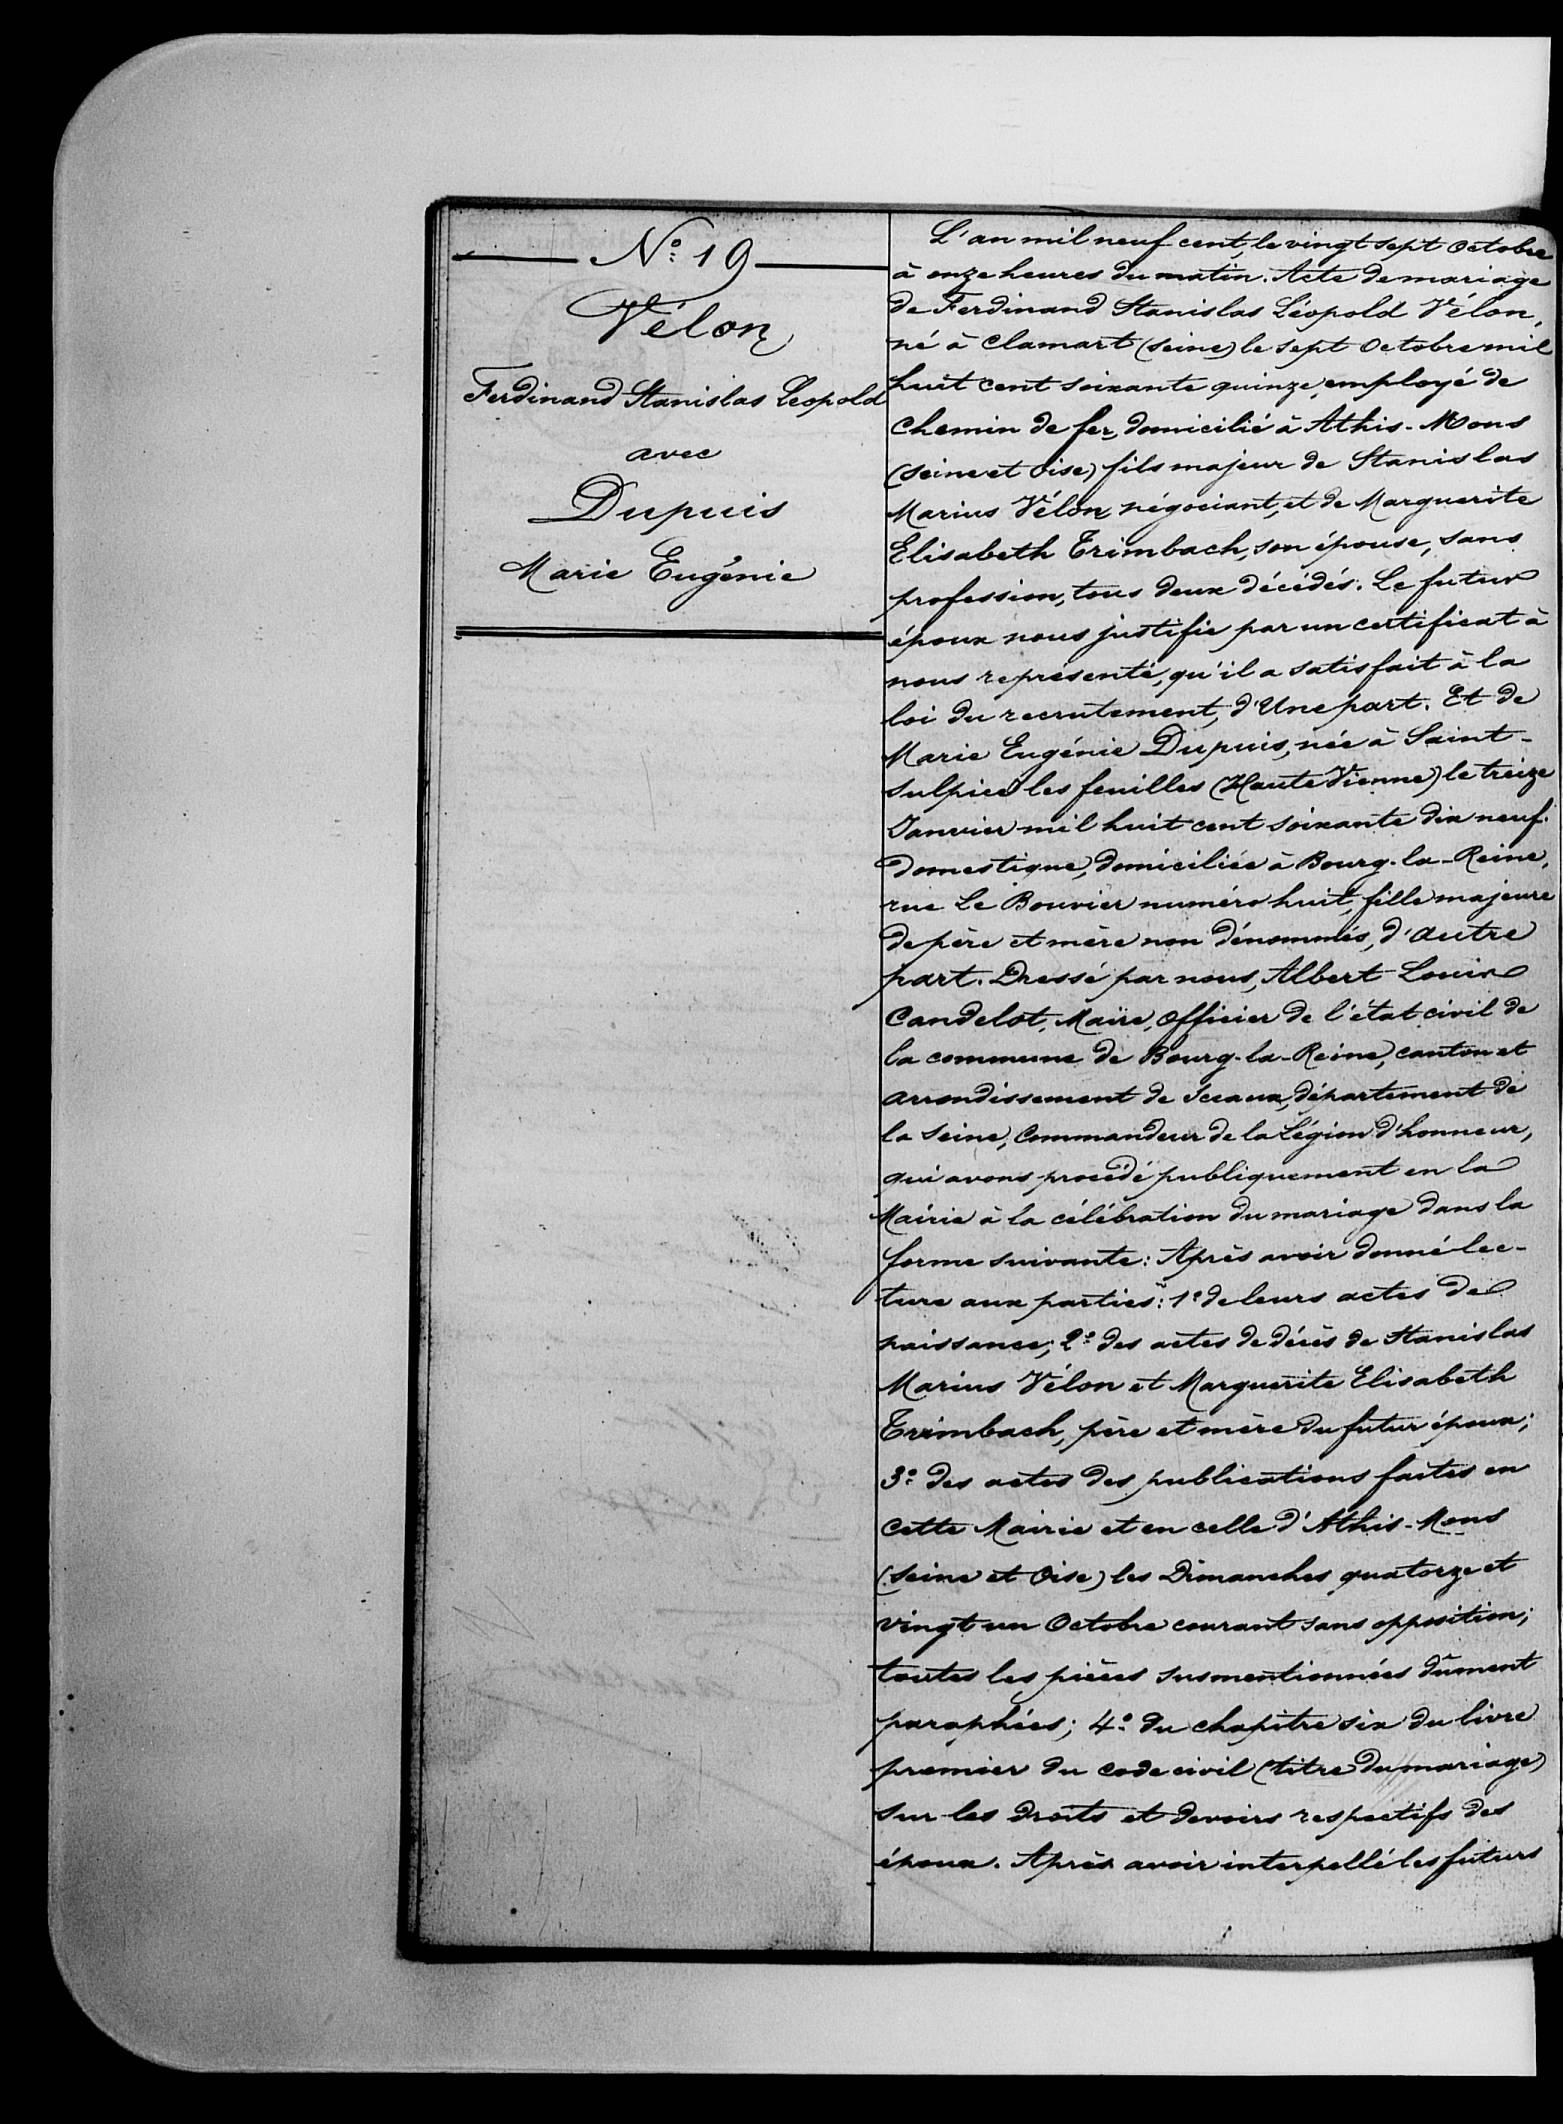N°19
Vélon
Ferdinand Stanislas Leopold
avec
Dupuis
Marie Eugénie
L'an mil neuf cent le vingt sept octobre
à onze heures du matin. Acte de mariage
de Ferdinand Stanislas Léopold Vélon,
né à Clamart (Seine) le sept octobre mil
huit cent soixante quinze employé de
chemin de fer domicilié à Athis-Mons
(Seine et Oise) fils majeur de Stanislas
Marius Vélon négociant, et de Marguerite
Elisabeth Trimbach, son épouse, sans
profession, tous deux décédés. Le futur
époux nous justifie par un certificat à
nous représenté, qu'il a satisfait à la
loi du recrutement d'une part. Et de
Maria Eugénie Dupuis, née à Saint-
sulpice les feuilles (Haute Vienne) le treize
janvier mil huit cent soixante dix neuf
domestique domiciliée à Bourg-la-reine,
rue le bouvier numéro huit fille majeure
de père et mère non dénommés, d'autre
part. Dressé par nous, Albert Louis
Candelot, Maire, Officier de l'état civil de
la commune de Bourg-la-Reine, canton et
arrondissement de Sceaux, département de
la Seine, commandeur de la Légion d'Honneur,
qui avons procédé publiquement en la
Mairie à la célébration du mariage dans la
forme suivante: Après avoir donné lec-
ture aux parties: 1° de leurs actes de
naissance; 2° des actes de décès de Stanislas
Marins Vélon et Marguerite Elisabeth
Trimbaud, père et mère du futur époux;
3° des actes des publications faites en
cette mairie et en celle d'Athis Mans
(Seine-et-Oise) les Dimanches quatorze et
vingt un Octobre courant sans opposition;
toutes les pièces susmentionnées dûment
paraphées; 4° du chapitre six du livre
premier du code civil (titre du mariage)
sur les droits et devoirs respectifs des
époux. Après avoir interpellé les futurs
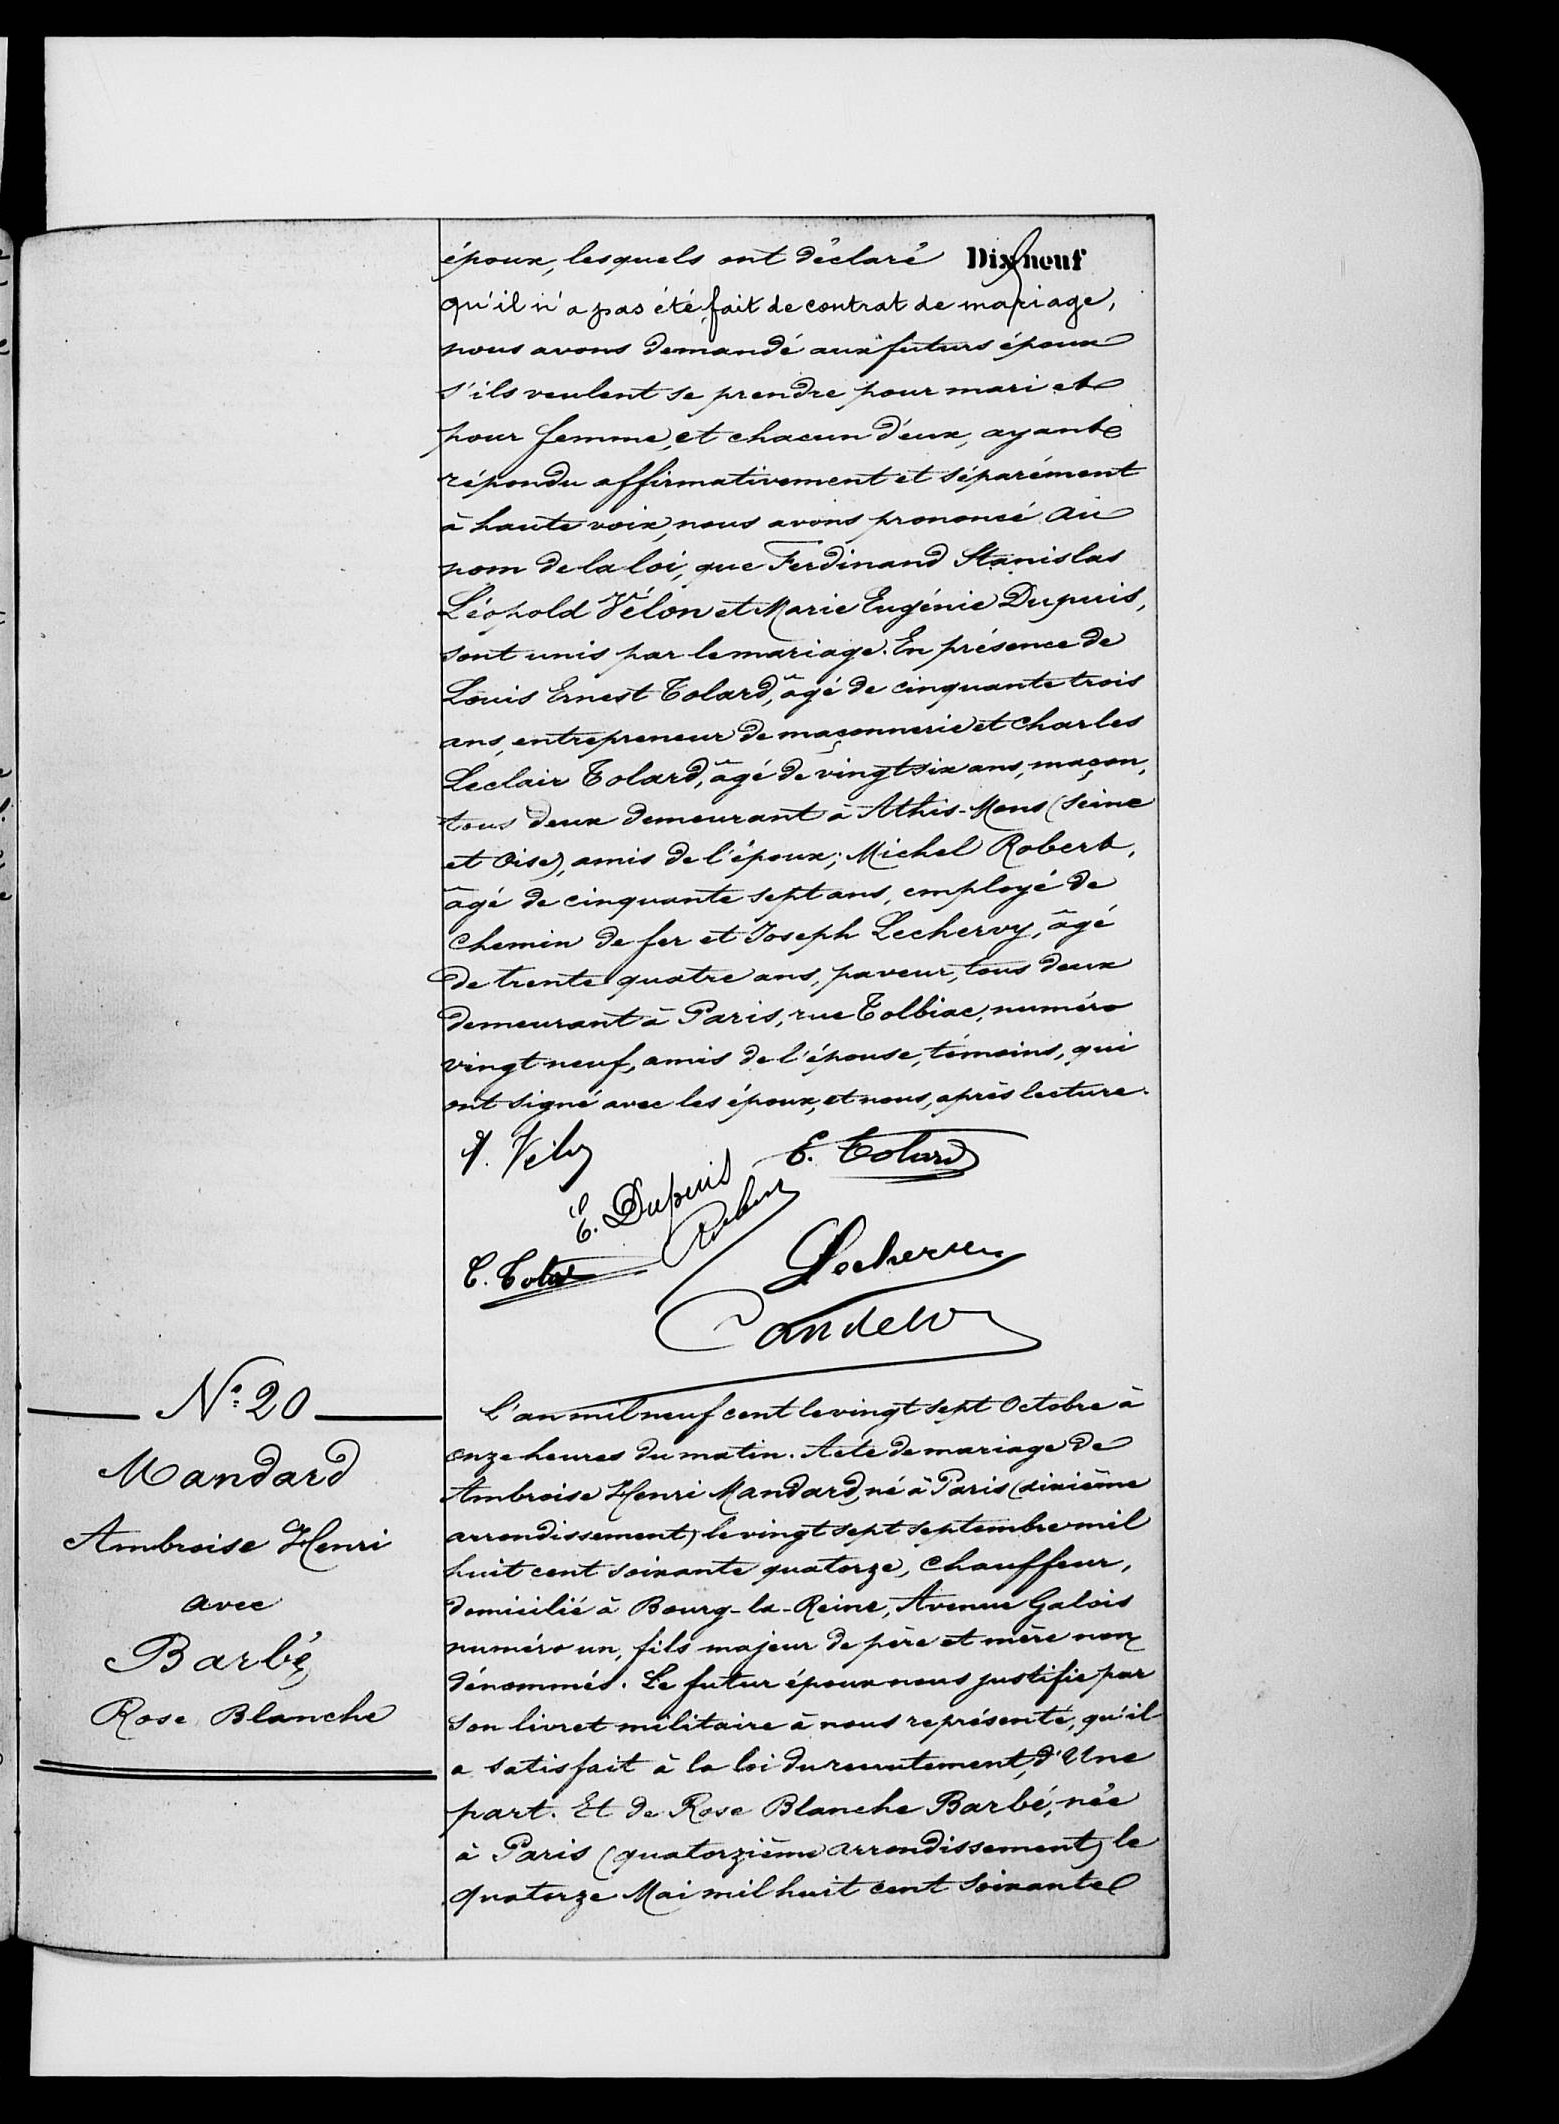époux, lesquels ont déclaré
qu'il n'a pas été fait de contrat de mariage
nous avons demandé aux futurs époux
s'ils veulent se prendre pour mari et
pour femme et chacun d'eux ayant
répondu affirmativement et séparément
à haute voix, nous avons prononcé au
nom de la loi, que Ferdinand Stanislas
Léopold Vélon et Marie Eugénie Dupuis,
sont unis par le mariage. En présence de
Louis Ernest Tolard, âgé de cinquante trois
ans, entrepreneur de maçonnerie et Charles
Leclair Tolard, âgé de vingt six ans, maçon,
tous deux demeurant à Athis-Mans (Seine
et Oise), amis de l'époux; Michel Robert,
âgé de cinquante sept ans, employé de
chemin de fer et Joseph Lechervy, âgé
de trente quatre ans, paveur, tous deux
demeurant à Paris, rue Tolbiac, numéro
vingt neuf, amis de l'épouse, témoins, qui
ont signé avec les époux, et nous, après lecture
N°20
Mandard
Ambroise Henri
avec
Barbé
Rose Blanche
L'an mil neuf cent le vingt sept octobre à
onze heures du matin. Acte de mariage de
Ambroise Henri Mandard, né à Paris (dixième
arrondissement) le vingt sept septembre mil
huit cent soixante quatorze, chauffeur,
domicilié à Bourg-la-Reine, Avenue Galois
numéro un, fils majeur de père et mère non
dénommé. Le futur époux nous justifie par
son livret militaire à nous représenté, qu'il
a satisfait à la loi du recrutement, d'une
part. Et de Rose Blanche Barbé, née
à Paris (quatorzième arrondissement) le
quatorze Mai mil huit cent soixante